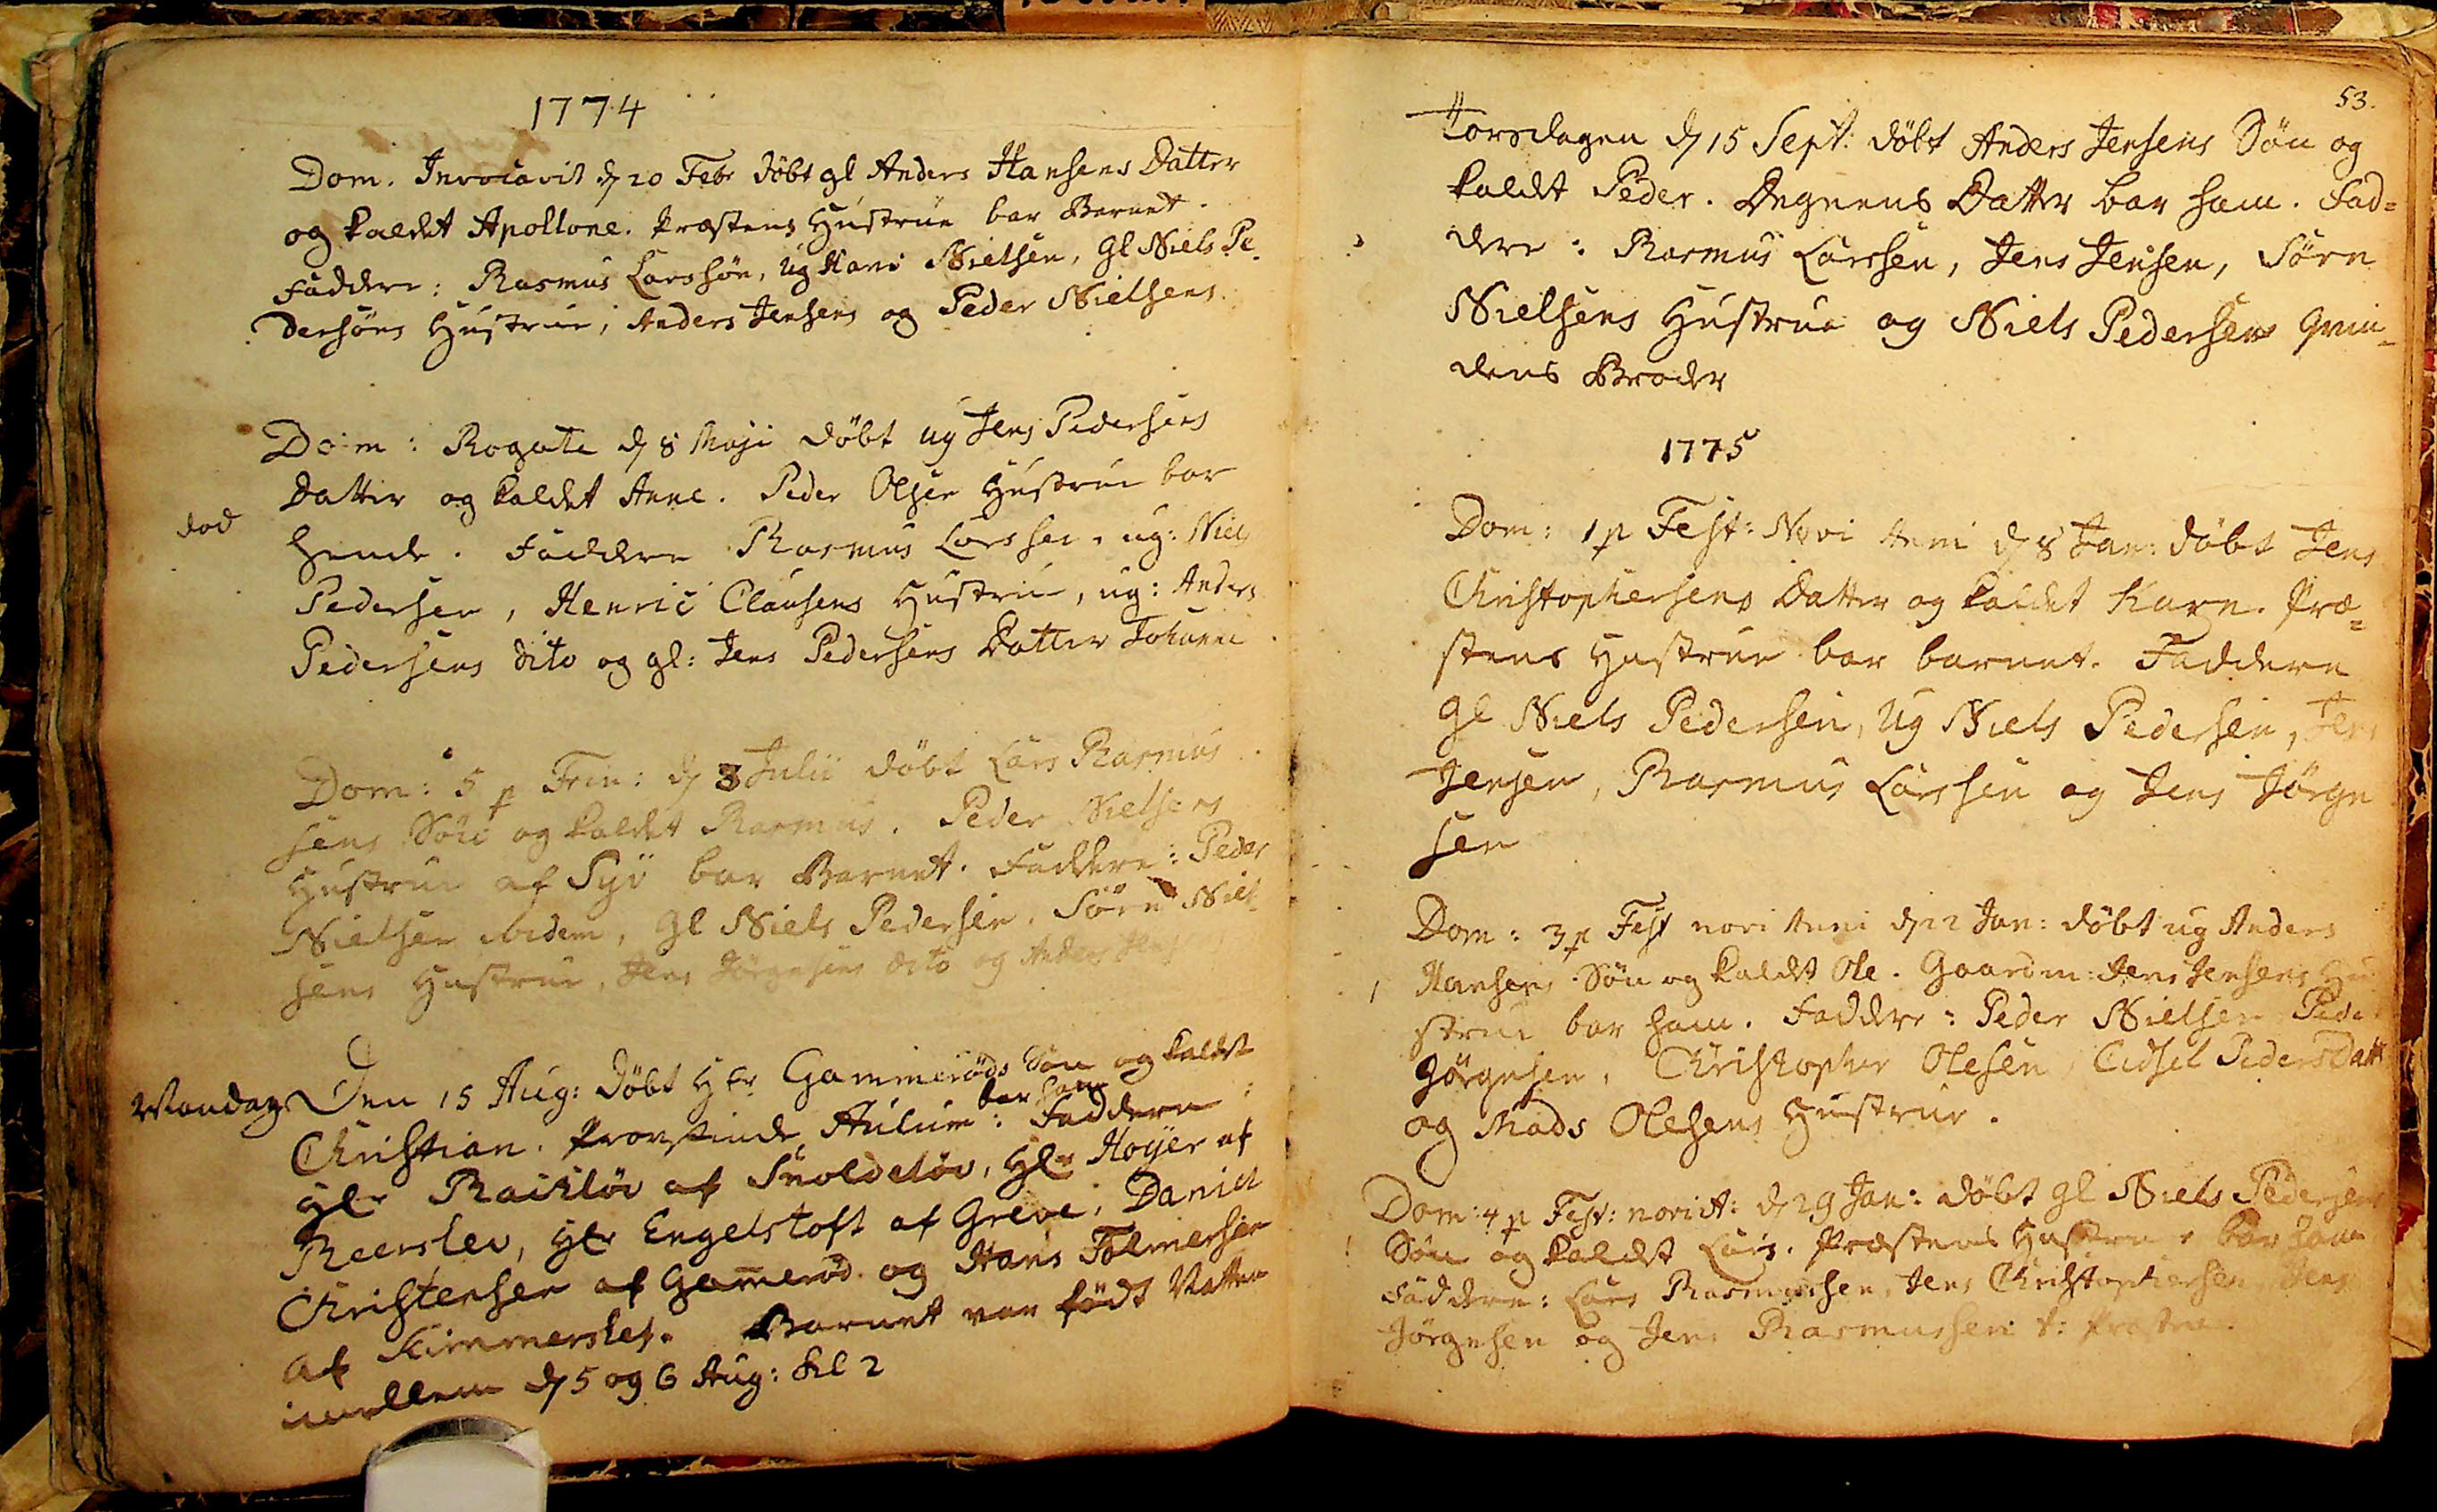1774.
Dom: Invocavit d 20 Febr døbt gl Anders Hansens Datter
og kaldet Apollone. Præstens Hustrue bar Barnet.
Faddere: Rasmus Larssøn, Ug Hans Nielsen, gl Niels Pe¬
dersøns Hustrue, Anders Jensens og Peder Nielsens.
Dom: Rogate d 8 Maji døbt ug Jens Pedersens
Datter og kaldet Anne. Peder Olsens Hustrue bar
død
hende. Faddere Rasmus Larssen. ug: Niels
Pedersen, Henric Clausens Hustrue, ug: Anders
Pedersens dito og gl: Jens Pedersens Datter Johanne.
Dom: 5 p Trin: d 3 Julii døbt Lars Rasmus
sens Søn og kaldet Rasmus. Peder Nielsens
Hustrue af Syv bar Barnet. Faddere: Peder
Nielsen ibidem, Gl Niels Pedersen, Sørn Niel¬
sens Hustrue, Jens Jørgnsens dito og Anders Jensens
Mandag Den 15 Aug: døbt Hr Gammerøds Søn og kaldet
bar ham
Christian. Provstinde Aulum Faddere:
Hr Rackløv af Snoldeløv, Hr Høyer af
Reerslev, Hr Engelstoft af Greve, Daniel
Christensen af Gammerød og Hans Folmersen
af Kimmerslef. Barnet var født Natten
imellem d 5 og 6 Aug: Kl 2
53.
Torsdagen d 15 Sept: døbt Anders Jensens Søn og
kaldt Peder. Degnens Datter bar ham. Fad¬
dere: Rasmus Larssen, Jens Jensen, Sørn
Nielsens Hustrue og Niels Pedersen qvin¬
dens Brodr
1775
Dom: 1 p Fest: Novi Anni d 8 Jan: døbt Jens
Christophersens Datter og kaldet Karn. Præ¬
stens Hustrue bar barnet. Faddere
gl Niels Pedersen, Ug Niels Pedersen, Jens
Jensen, Rasmus Larssen og Jens Jørgn
sen
Dom: 3 p Fest novi Anni d 22 Jan: døbt ug Anders
Hansens Søn og kaldt Ole. Gaardm: Jens Jensens Hu
strue bar ham. Faddere: Peder Nielsen, Peder
Jørgnsen, Christopher Olesen, Cidsel Pedersdatter
og Mads Olesens Hustrue.
Dom: 4 p Fest: novi A: d 29 Jan: døbt gl Niels Pedersens
Søn og kaldt Lars. Præstens Hustrue bar ham.
Faddere: Lars Rasmussen, Jens Christophersen, Jens
Jørgnsen og Jens Rasmussen t: Præsten. 
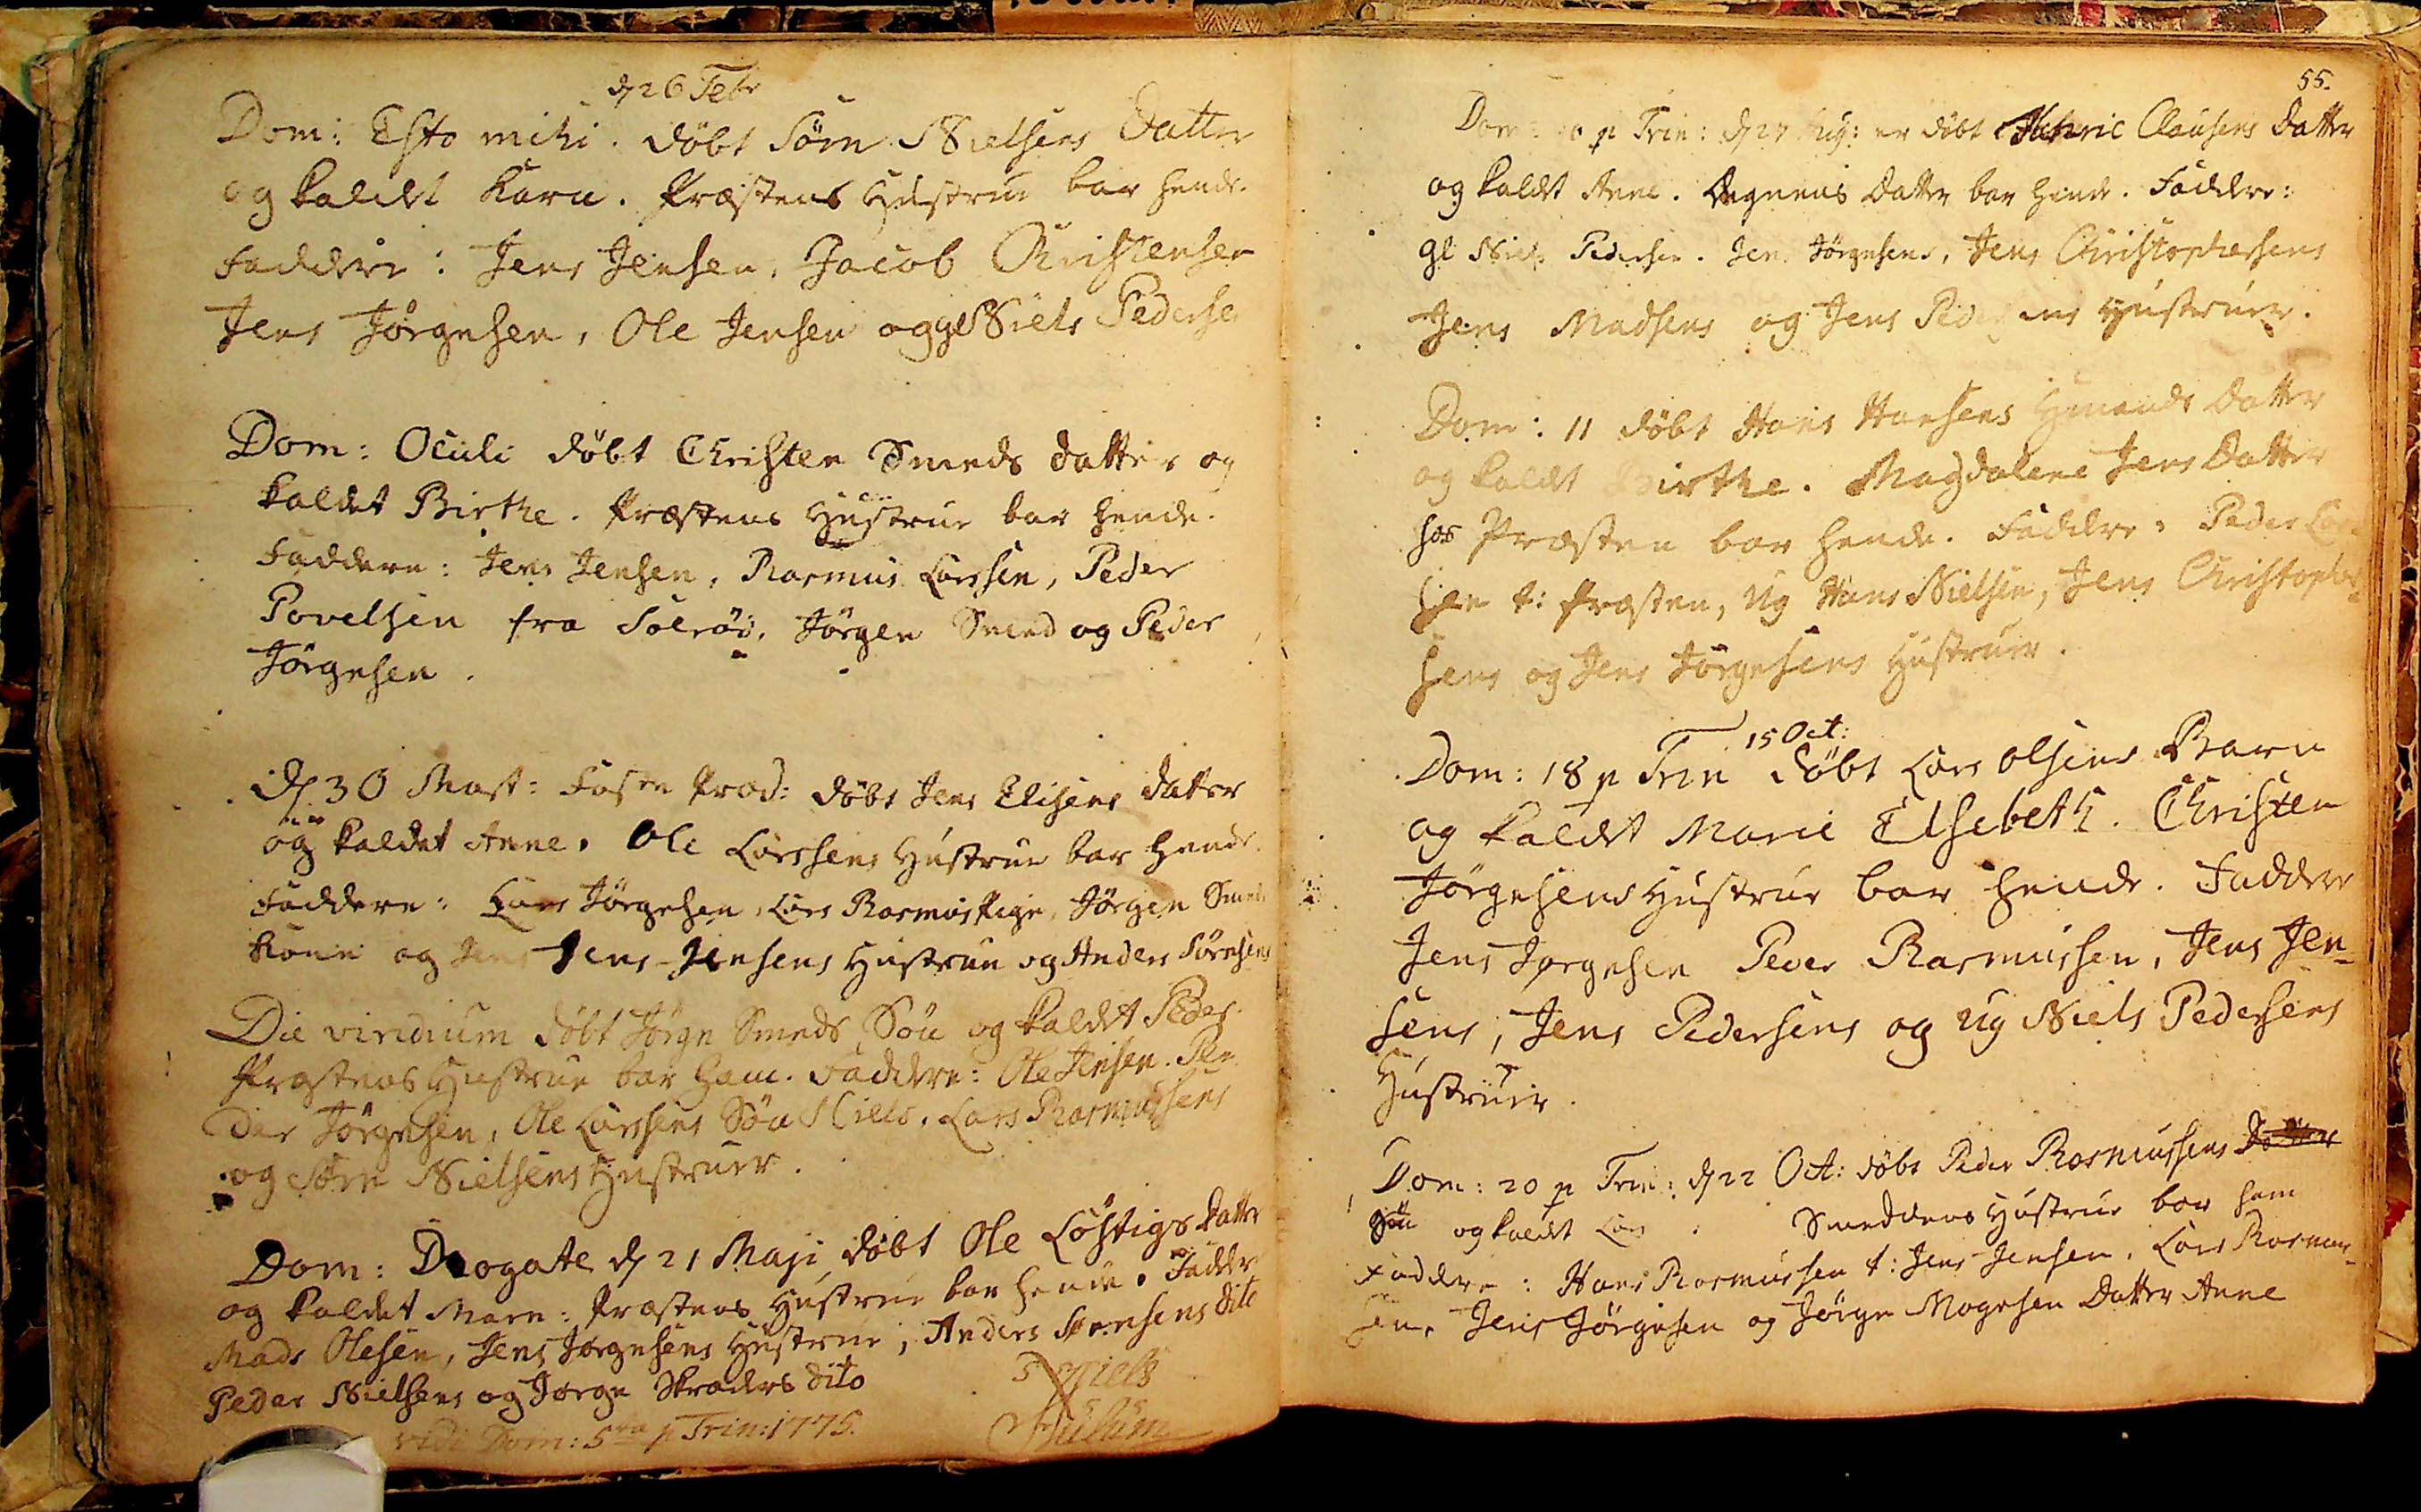d 26 Febr
Dom: Esto mihi. døbt Sørn Nielsens Datter
og kaldt Karn. Præstens Hustrue bar hende.
Faddere: Jens Jensen, Jacob Christensen
Jens Jørgnsen, Ole Jensen og gl Niels Pedersen
Dom: Oculi døbt Christen Smeds datter og
kaldet Birthe. Præstens Hustrue bar hende.
Faddere: Jens Jensen, Rasmus Larssen, Peder
Povelsen fra Solrød, Jørgen Smed og Peder
Jørgnsen
d. 30 Mart: Faste Præd: døbt Jens Elisens datter
og kaldet Anne. Ole Larssens Hustrue bar hende.
Faddere: Lars Jørgnsen, Lars Rasmus Pige, Jørgen Smeds
Kone og Jens Jens Jensens Hustrue og Anders Sørnsens
Die viridium døbt Jørgn Smeds Søn og kaldt Peder.
Præstens Hustrue bar ham. Faddere: Ole Jensen. Pe¬
der Jørgnsen, Ole Larssens Søn Niels. Lars Rasmussens
og Sørn Nielsens Hustruer.
Dom: Rogate d 21 Maji, døbt Ole Løstigs Datter
og kaldet Marn: Præstens Hustrue bar hende. Faddere
Mads Olesen, Jens Jørgnsens Hustrue, Anders Sørnsens dito
Peder Nielsens og Jørgn Skræders dito
vidi Dom: 5ta p Trin 1775.     
Niels
Aulum
55.
Dom: 10 p Trin: d 27 Aug: er døbt Henric Clausens Datter
og kaldt Anne. Degnens Datter bar hende. Faddere:
gl Niels Pedersen. Jens Jørgnsens, Jens Christophersens
Jens Madsens og Jens Pedersens Hustruer.
Dom: 11 døbt Hans Hansens Hmands Datter
og kaldt Birthe. Magdalene Jens Datter
hos Præsten bar hende. Faddere: Peder Lar
sen t: Præsten, Ug Hans Nielsen, Jens Christopher
sens og Jens Jørgnsens Hustruer.
15 Oct:
Dom: 18 p Trin døbt Lars Olsens Barn
og kaldt Marie Elsebeth. Christen
Jørgnsens Hustrue bar hende. Faddere
Jens Jørgnsen Peder Rasmussen, Jens Jen¬
sens, Jens Pedersens og Ug Niels Pedersens
Hustruer.
Dom: 20 p Trin: d 22 Oct: døbt Peder Rasmussens Datter
Søn og kaldt Lars. Smeddens Hustrue bar ham
Faddere: Hans Rasmussen t: Jens Jensen, Lars Rasmus¬
sen, Jens Jørgnsen og Jørgn Mognsen Datter Anne